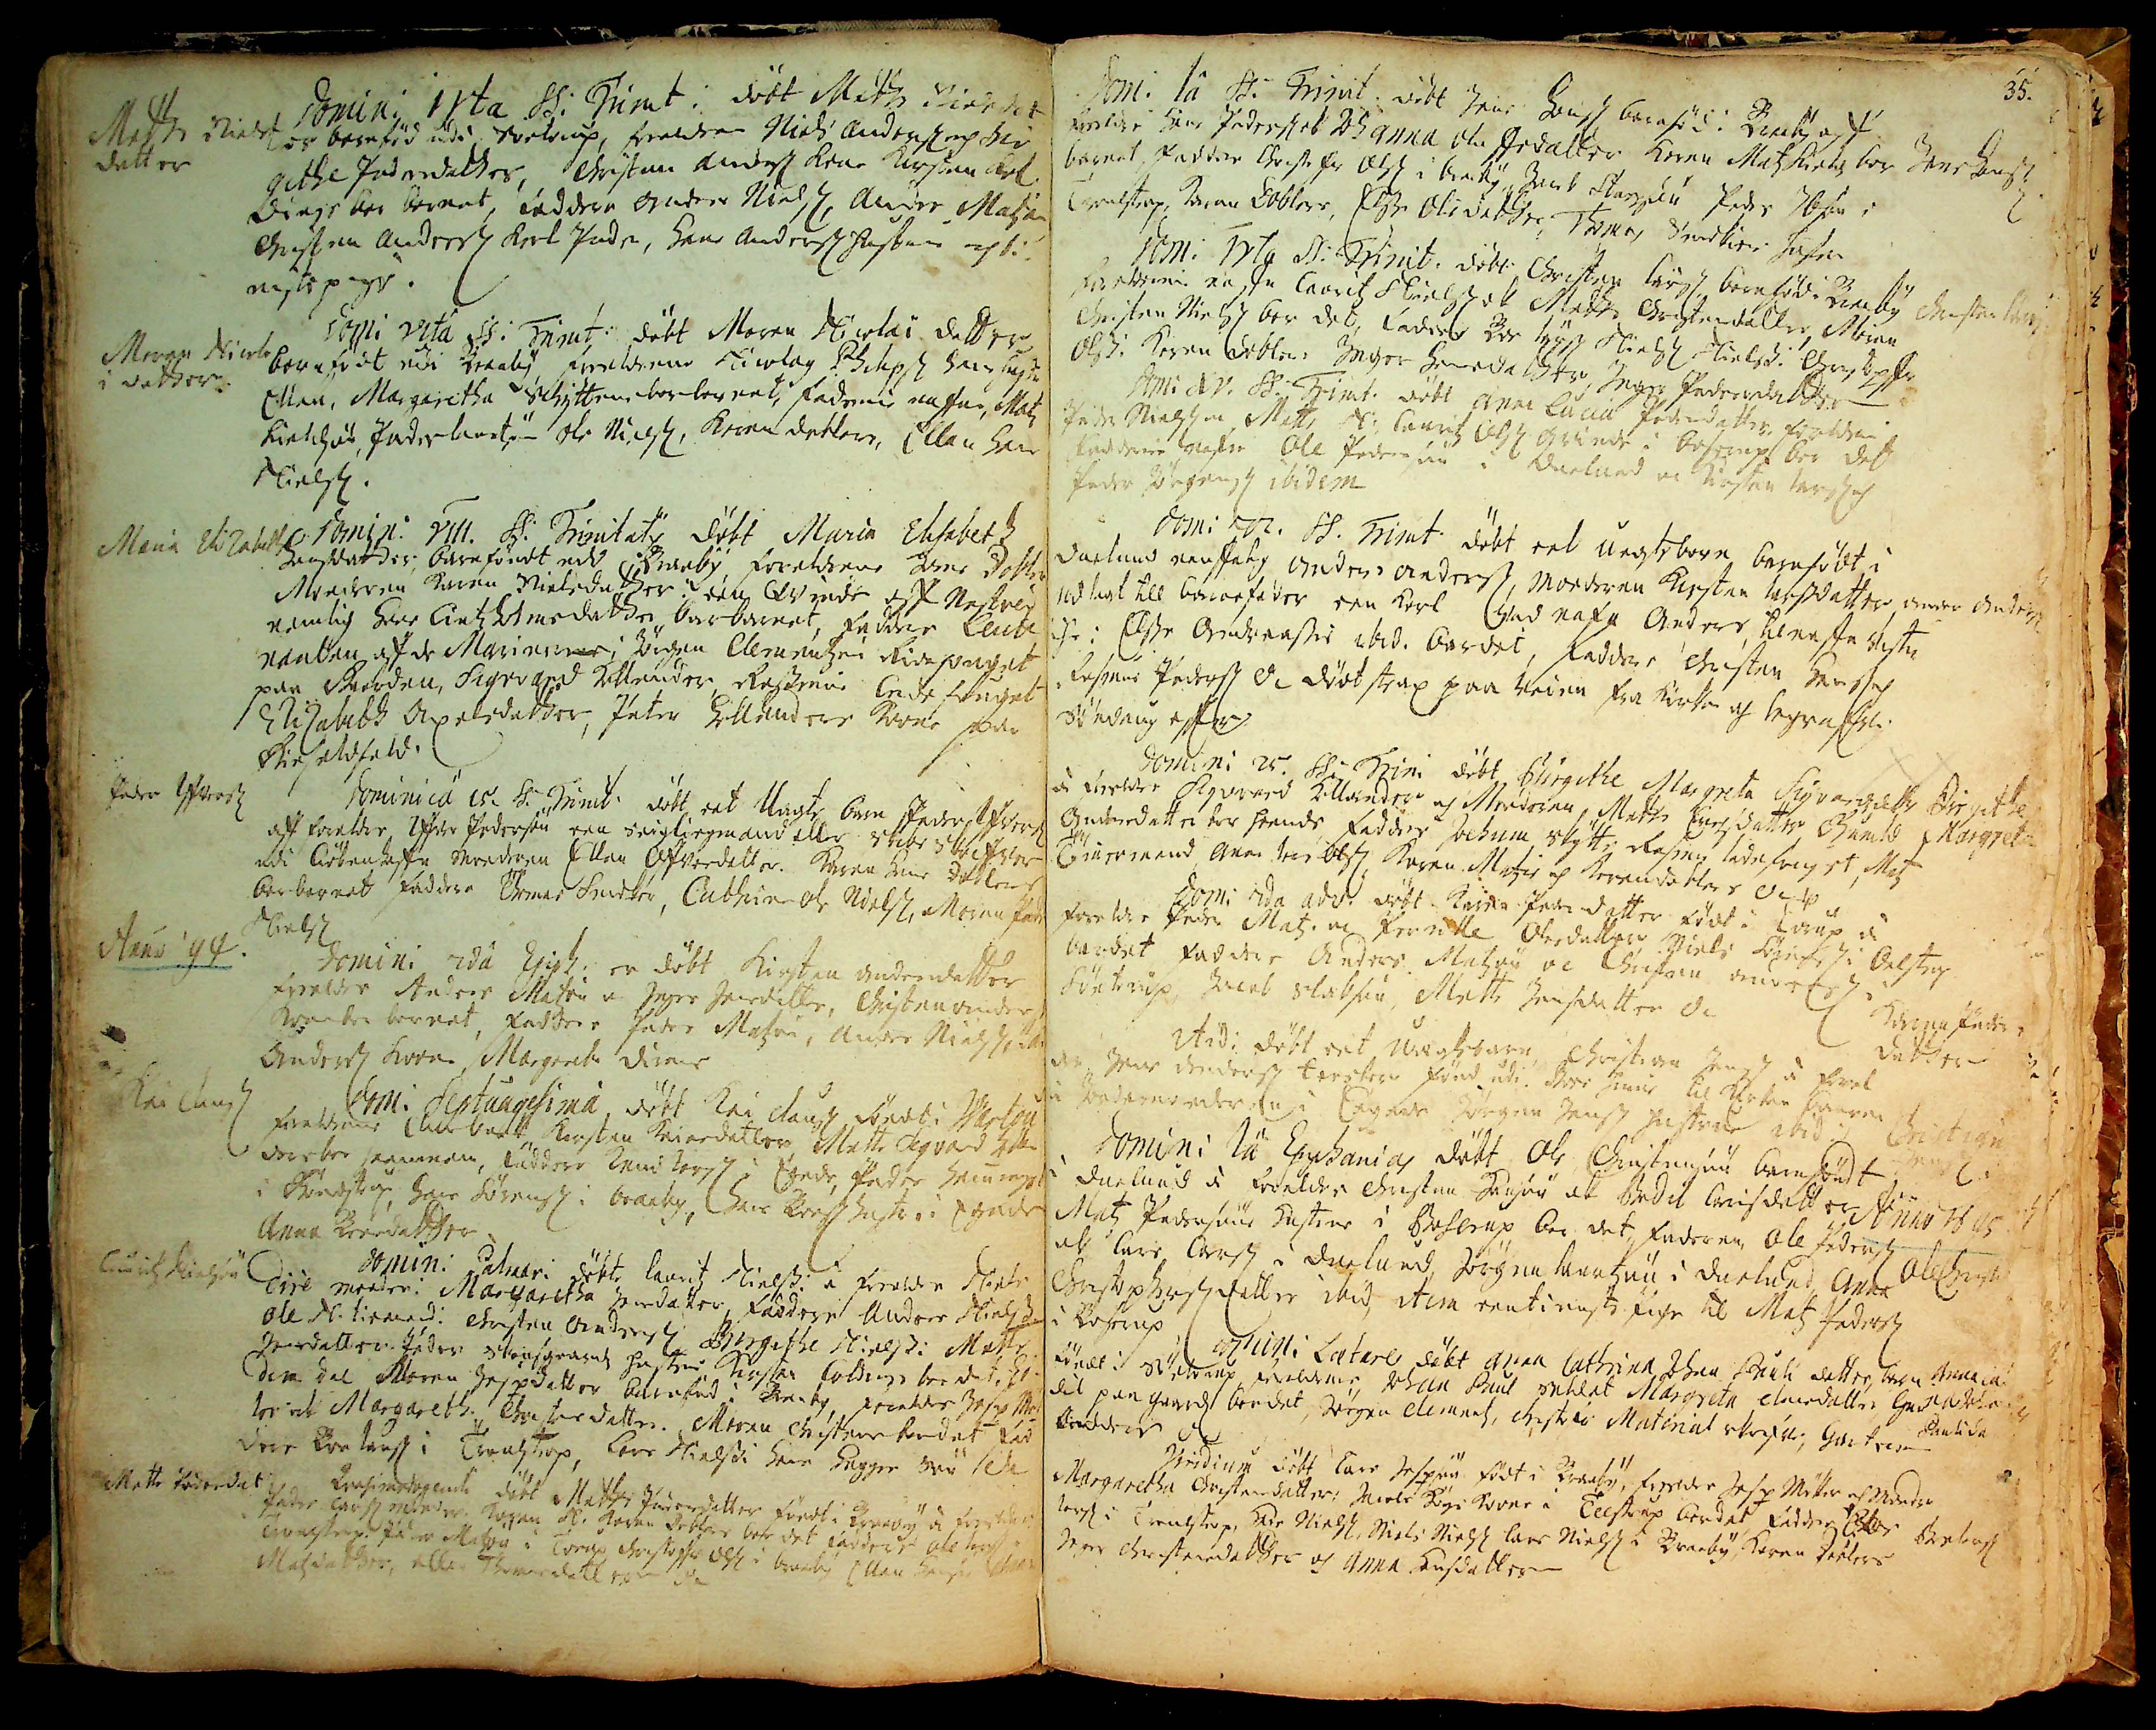Mette Niels
datter 
Domini IV ta SS: Trinit: døbt Mette Nielsdat
ter barnfød udi Søetorup, forældre Niels Anders och Bir
githe Pederdatter, Christen Anderss kone Kirsten Col
dings bar barnet, Faddere Anders Niels, Ander Matzøn,
Christen Anderss Karl Peder, Hans Anderss Hustrue ock ti
enistepige.
Maren Nicola
i datter
Domi VI ta SS: Trinit: døbt Maren Nicolai datter
barnfødt udi Braaby, foreldrene Nicolay Philips hans hust.
Ellen, Margaretha Schyttens bar barnet, Fadenis naffne Matz
Kieldsøn, Peder Laustzøn, Ole Niels, Karen Doblers, Ellen Hans
Nielss.
Maria Elizabeth
Domin: VIII. SS Trinitaty døbt Maria Elisabeth
Hansdatter, barn føedt udi Braaby, forældene Hans Dobler,
Moederen, Karen Nielsdatter, een Qvinde aff Nestved
nemlig Hans Amtzholms datter bar barnet, Fadder Leute
nanten aff de Marinenere; Jørgen Clementzen Ridefoeget
paa Gaarden, Sigvard Hollender, Rassmus Ladefouget, 
Elizabeth Axelsdatter, Peter Hollenders Kone paa 
Gisselfeld. 
Peder Iffvers
Dominica 15. SS Trinit. døbt eet uegte barn Peder Iffvers
aff forældre, Iffver Pedersøn een Seigliegmand eller Skibs Skrifver
udi Ciøbenhaffn, Moederen Ellen Iffversdatter. Karen Hans Doblers
bar barnet, Faddere Thomas Snidker, Cathrin Ole Nielss, Maren Peder
Niels.
Anno 94. 
Domini 2da Epiph: er døbt Kirsten Andersdatter
Forelder Anders Matzøn oc Jnger Jensdatter, Christen Anders
Kone bar barnet, Faddere Peder Matzøn, Anders Nielsøn, ##
Anders kone, Margrete Dines.
Kai Clauss
Dom: Septuagesima døbt Kai Clauss, føedt i Wartou
forælderne Claus Busk, Kirsten Kriesdatter. Mette Sigvard Hollen
ders bar hannem, Faddere Knud Lars i Egede, Peder Hemmings
i Giødstrup, Hans Sørenss i Braaby, Hans Boess Hustrue i Egede,
Anne Boesdatter.
Lauritz Nielsen
Domin Palmar: døbt Lauritz Nielss: a Forældre Niels
Dine. Moeder: Margaretha Hansdatter, Faddere Anders Niels,
Ole N. tinend: Christen Anderss, Birgithe Nielsd: Mette
Jensdatter. Peder Skoufgaards Hustrue, Kirsten Coldings bar det. Eo
dem Die Maren Jespdatter barnføed i Braaby. Forældre Jesp Mo##
ten ok Margareth. Christensdatter. Maren Christens bar det. Fad
dere: Boe Larss i Troelstrup, Lars Nielss, Hans Huggers Søn ##
Mette Pedersdat.
Quasimodogeniti døbt Mette Pedersdatter føedt i Braaby af Forældre:
Peder Larss, Moeder Karen N., Karen Doblers bar det Faddere Ole Larss i
Troelstrup. Peder Matzøn i Torup Christoffer Ols i Braaby, Ellen Hanss ## ##
Matzdatter, Ellen Thomasdatter ##
Dom 1a SS. Trinit. døbt Jens Hanss, barnfød i Braaby af
foreldre Hans Pederss ok Johanna Oluffsdatter. Karen Matz Kirks bar
barnet, Faddere Christofer Olss i Braaby. Jacob Stasxsøn, Peder Ibsøn i
Troelstrup, Karen Doblers, Else Olsdatter, Thomas Snedkirs Hustrue.
35.
Jens Hans
Dom: 13te SS: Trinit. døbt Christen Larss barnfød i Braaby,
foreldrenes nafn Lauritz Nielss ok Mette Christensdatter, Maren
Christen Nielss bar det, Fadere Boe ## Niels Niels: Christopfr
Olss: Karen Doblers, Inger Hansdatter, Inger Pedersdatter.
Christen Lars
Domi: XV. SS. Trinit: døbt Anna Lucia Pedersdatter, Foreldre
Peder Nielss oc Mette N. Lauritz Olss Qvinde i Boserup bar det,
Faddere Nafn. Ole Pedersøn i Duelund oc Kristen Larss och
Peder Jørgenss ibidem.
Dom: 22. SS. Trinit. døbt eet uegte barn barnfødt i
Duelund nauffnlig Anders Anderss, Moederen Kirsten Larsdatter,
udlagt till barnefader een Karl ved nafn Anders tienste Skyte
##: Else Andreasis ibid bar det. Faddere. Christen Hanss,
Rasmus Pederss, er dødt strax paa Veien fra Kirken och begraffet
Søndaug effter.
Anders Anderss
Domini 25. SS Trin: døbt Birgithe Magreta Sigvardsdatter
à forældre Sigvard Hollender och Moederen Mette Erichsdatter, Gunild
Andersdatter bar hende, Faddere Jochum Skytte, Rasmus Ladefouget, Matz
Tienermand Ane Jens Olss, Karen Matzis och Karen Doblers ###.
Birgithe 
Margreta
Dom: 2da advt. døbt Karen Pedersdatter født i Torup à
forældre Peder Matz. oc Pernille Olesdatter, Niels Christens i Oelstrup
bar det. Faddere: Anders Matzøn oc Christen Anderss i
Søetoerup, Jacob Staksøn, Mette Jensdatter.
Karen Peders
datter
Itid: døbt eet Uægte barn, Christian Jensøn à forel
dre Jens Anders, Tersker. Føed udi Boes Huus til Kirken, baaren
a Jordemoederen i Eegede, Jørgen Jensøns hustrue ibid:
Christian
Jens
Domini 1a Epiphania døbt Ole Chrisensøn barn føedt
i Duelund à forældre Christen Hansøn ok Bodil Larsdatter,
Matz Pedersøns Hustrue i Boserup bar det, Fadere, Ole Peders
ok Lars Lars i Duelund, Jørgen Laurtzøn i Duelund, Anna
Christophersdatter ibid. item een tienste Pige til Matz Peders
i Boeserup.
Anno 1695
Ole Christens
Domini Lætære døbt Anna Catrine Johan Pauli datter, barn
føedt i Søetorup, Forældre Johan Paul soldat, Margreta Clausdatter, Ger
dit paa gaarden bar det, Jørgen Clements, Christen Material Skrifver, Gartner
Erichsens.
Anna Cat
rina ##
Paulida##
Viridium døbt Lars Jespsøn født i Braaby. Forælder, Jesp Møller och moeder
Margretha Christensdatter: Jacobs Pige Karen i Teestrup bar det, Faddere, Lars
Lars i Troelstrup, Hans Niels, Niels Niels, Lars Niels i Braaby. Karen Doblers,
Inger Christensdatter och Anna Hansdatter.
Lars Ieps